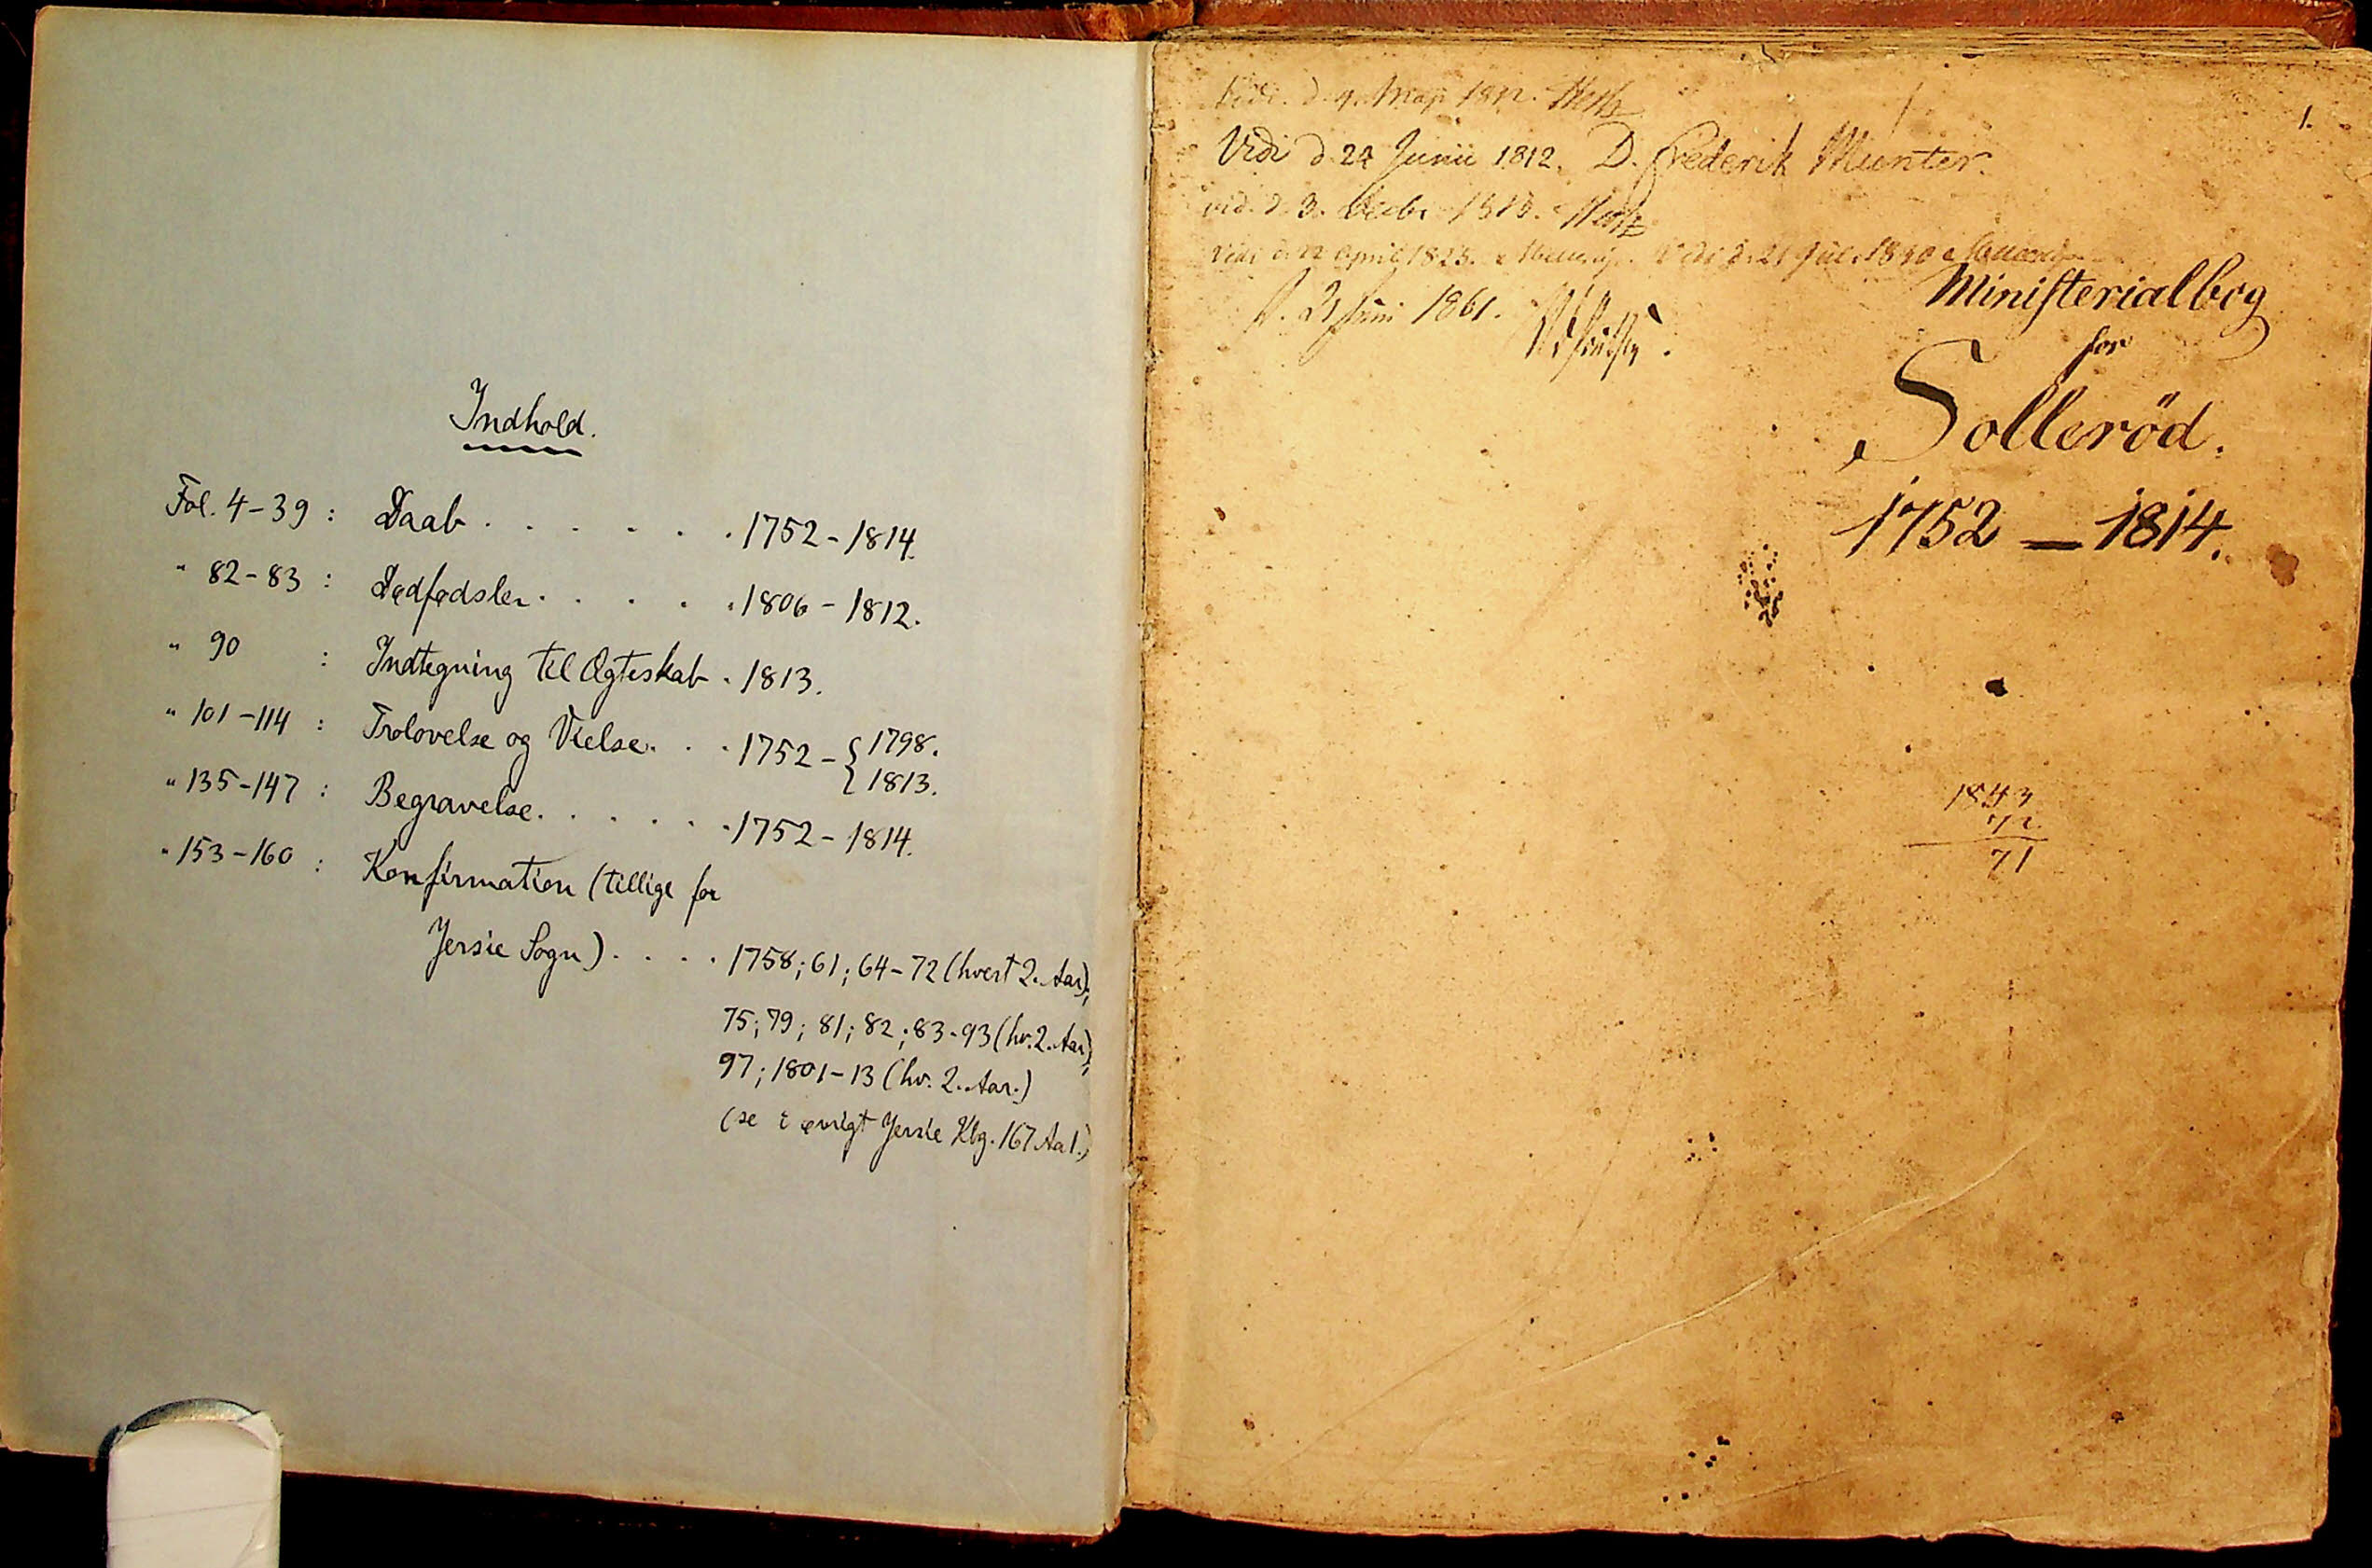Indhold.
Fol. 4-39 : Daab.  .  .  .  .  . 1752-1814.
82-83 : Dødfødsler.  .  .  .  . 1806-1812.
90  : Indtegning til Ægteskab. 1813.
101-114 : Trolovelse og Vielse .  .  . 1752 -
1798.
1813.
135-147 : Begravelse.  .  .  .  .  . 1752-1814.
153 - 160 : Konfirmation (tillige for 
Jersie Sogn)
1758; 61; 64-72 (hvert 2. Aar);
75; 79; 81; 82; 83-93 (hv.2.Aar),
97; 1801-13 (hv.2.Aar.)
(se i øvrigt Jersie Kbg.167 Aa1.)
Vidi. d. 4. Maji 1812. Hertz
Vidi. d. 24 Junii 1812. D. Frederik Münter.
vid. d. 3. Decb: 1813. Hertz
Vidi d: 22 April 1825. Mellerup. Vidi d: 21 Jul: 1830 Mellerup##
D. 21 Juni 1861.
W## Poulsen
Ministerialbog
for
Sollerød.
1752-1814
1843
72
71